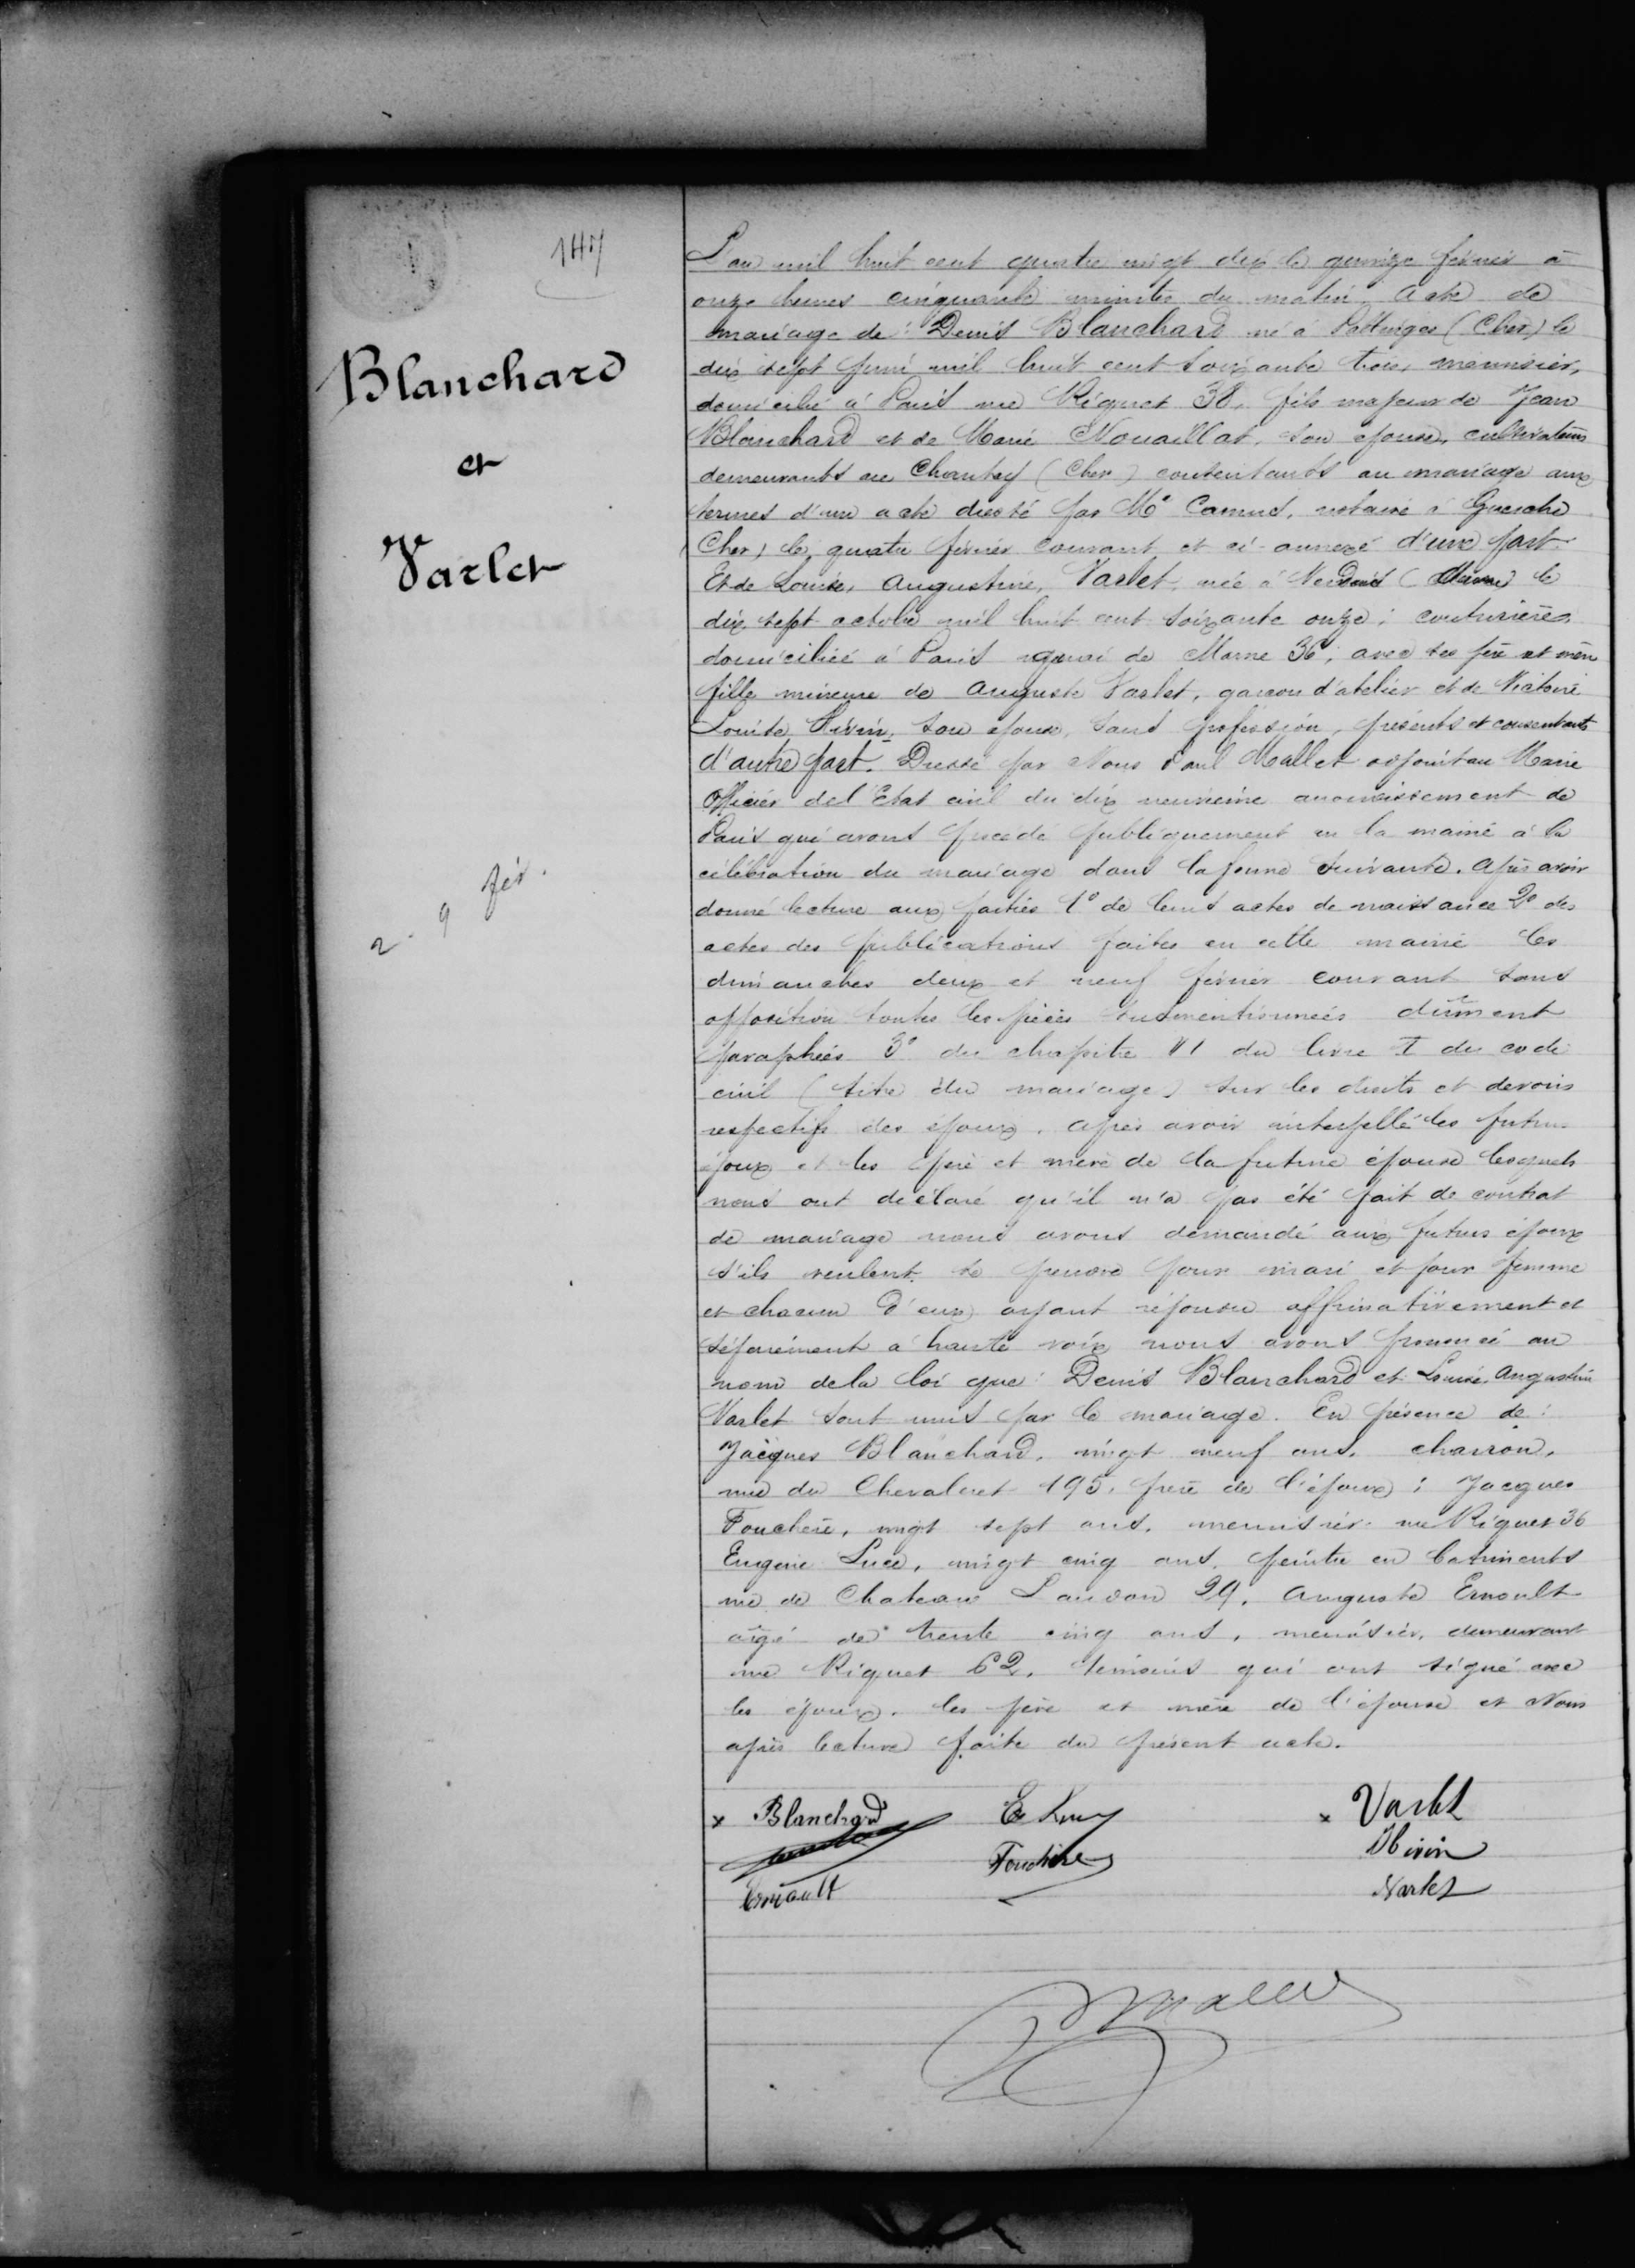147
Blanchard
et
Varlet
L'an mil huit cent quatre vingt dix le quinze février à
onze heures cinquante minutes du matin. Acte de
mariage de Denis Blanchard né à Pattunges (Cher) le
dix sept juin mil huit cent soixante trois, menuisier,
domicilié à Paris rue Réquet 38. fils majeur de Jean
Blanchard et de Marie Nouallat, son épouse, cultivateurs
demeurant au Chantey (Cher) consentants au mariage aux
termes d'un acte dressé par Me Camud, notaire à Guesche
(Cher) les quatre février courant et si-annexé d'une part.
Et de Louise, Augustine, Varlet, née à Nerdand (Marne) le
dix sept octobre mil huit cent soixante onze: couturière
domiciliée à Paris quai de Marne 36; avec ses père et mère
fille mineure de Auguste Varlet, garçon d'atelier et de Victoire
Louise Hivin, son époux, sans profession, présents et consentants
d'autre part. Dressé par Nous Paul Mallet adjoint au Maire
Officier de l'Etat civil du dix neuvième arrondissement de
Paris qui avons procédé publiquement en la mairie à la
célébration du mariage dans la forme suivante. après avoir
donné lecture aux parties 1° de leurs actes de naissance 2° des
actes de publications faites en cette maire les
dimanches deux et neuf février courant sans
opposition toutes les pièces susmentionées dûment
paraphées 3° du chapitre VI du livre 1 du code
civil (titre du mariage) sur les droits et devoirs
respectifs des époux. après avoir interpellé les futurs
poux et les père et mère de la future épouse lesquels
nous ont déclaré qu'il n'a pas été fait de contrat
de mariage nous avons demandé aux futurs époux
s'ils veulent se prendre pour mari et pour femme
et chacun d'eux ayant répondu affirmativement et
séparément à haute voix nous avons prononcé au
nom de la loi que: Denis Blanchard et Louise Augustine
Varlet sont unis par le mariage. En présence de:
Jacques Blanchard, vingt neuf ans, charron,
rue du Chevaleret 195,frère de l'épouse: Jacques
Fouchère, vingt sept ans, menuisier, rue Riquet 36
Eugénie Luce, vingt cinq ans, peintre en batiments
rue du Chateau Laudan 29, Auguste Ernoult
âgé de trente cinq ans, menuisier, demeurant
rue Riquet 62, témoins qui ont signé avec
les époux, les père et mère de l'épouse et Nous
après lecture faite du présente acte.
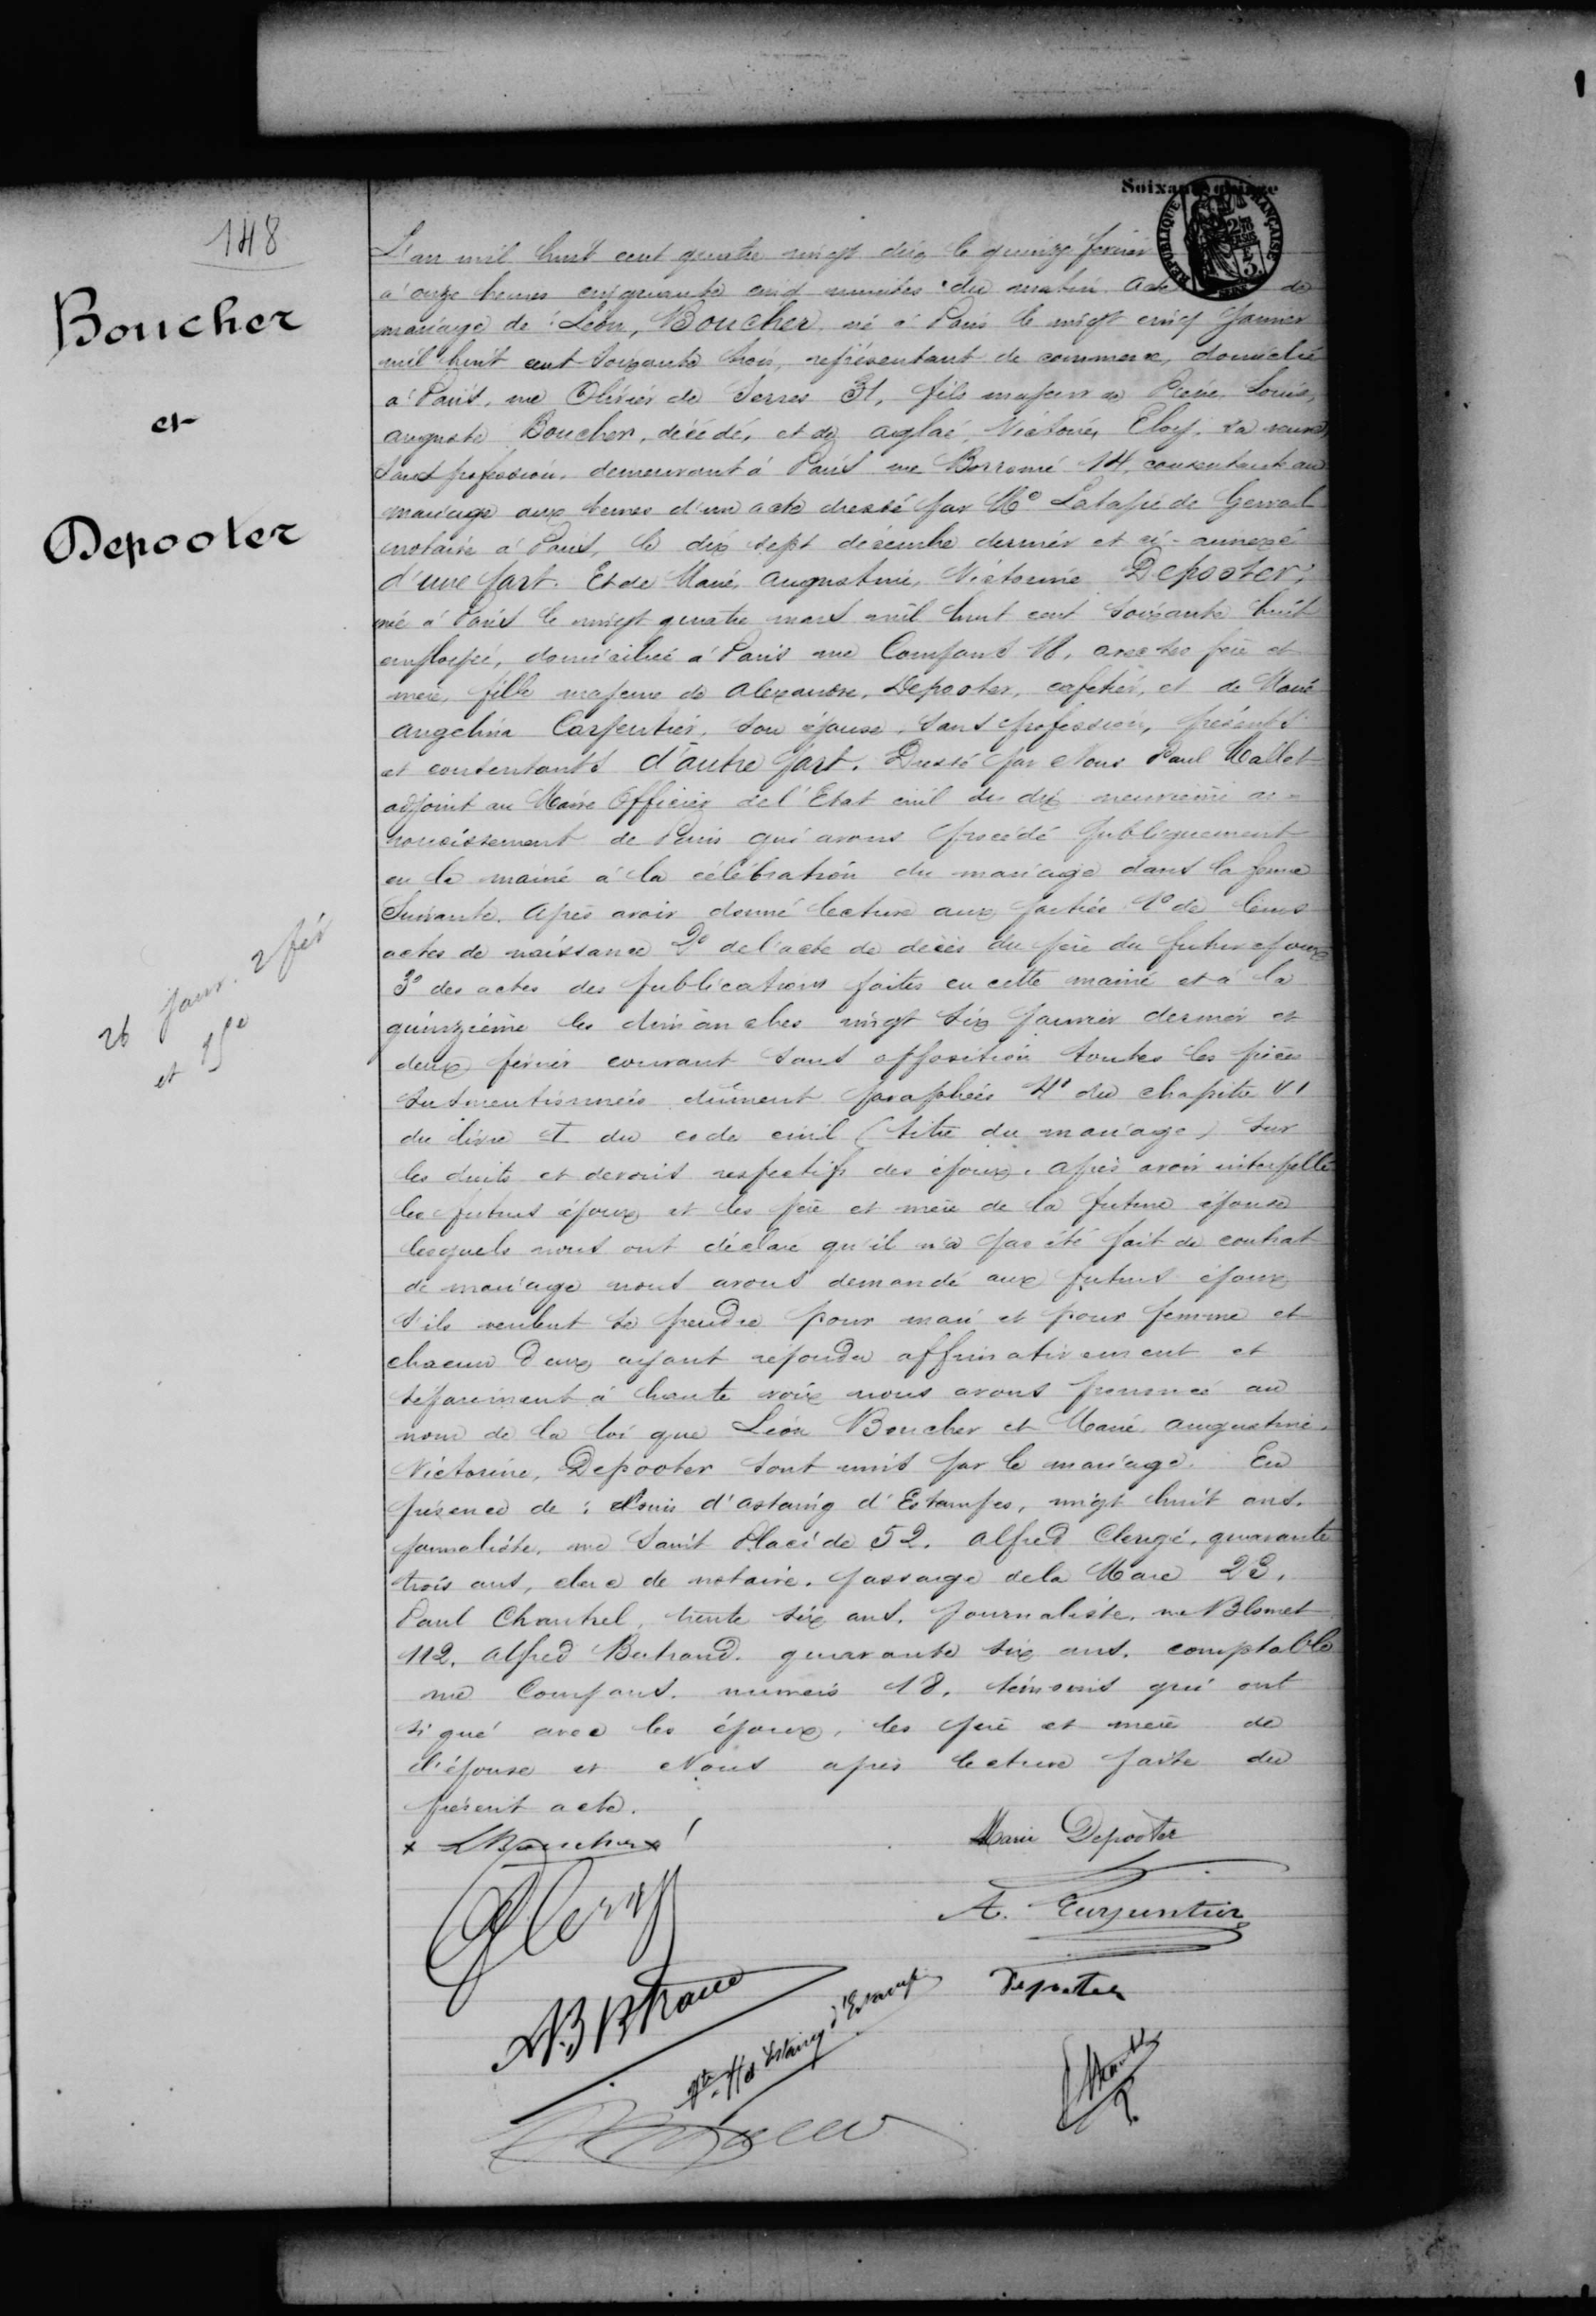148
Boucher
et
Depooler
L'an mil huit cent quatre vingt dix le quinze février
à onze heures cinquante cinq minutes du matin acte de
mariage de Léon, Boucher, né à Paris le vingt cinq Janvier
mil huit cent soixante trois, représentant de commerce, domicilié
à Paris, rue Olivier de Serres 31, fils majeur de René Louis,
auguste Boucher, décédé, et de aglaé, Victoire Eloy, sa veuve,
sans profession, demeurant à Paris rue Borroné 14, consentant au
mariage aux heures d'un acte dressé par M° Latapi de Gerval
notaire à Paris le dix sept décembre dernier et ci-annexé
d'une part. Et de Marie, Augustine, Victorine, Depooler,
né à Paris le vingt quatre mars mil huit cent soixante huit
employé, domiciliée à Paris rue Confand 18, avec ses père et
mère, fille majeure de Alexandre Depooler, cafetier, et de Nous
angelina Carpentier, son épouse, sans profession, présents
et consentants d'autre part. Dressé par Nous Paul Mallet
adjoint au Maire Officier de l'Etat civil du dix-neuvième ar-
rondissement de Paris qui avons procédé publiquement
en la mairie à la célébration du mariage dans la forme
suivante. après avoir donné lecture aux parties 1° de leurs
actes de naissance 2° de l'acte de décès du père du futur epoux
3° des actes des publications faites en cette mairie et à la
quinzième les dimanches vingt six janvier dernier et
deux février courant sans opposition toutes les pièces
susmentionnées dûment paraphées 4° du chapitre VI
du livre I du code civil (titre du mariage) sur
les droits et devoirs respectifs des époux. après avoir interpellé
les futurs époux et les père et mère de la future épouse
lesquels nous ont déclaré qu'il n'a pas été fait de contrat
de mariage nous avons demandé aux futurs époux
s'ils veulent se prendre pour mari et pour femme et
chacun d'eux ayant répondu affirmativement et
séparément à haute voix nous avons prononcé au
nom de la loi que Léon Boucher et Marie augustine
Victoire, Depooler sont unis par le mariage. En
présence de: Louis d'astaing d'Estampes vingt huit ans,
journalier, rue Saint Placide 52. Alfred Clengé, quarante
trois ans, clerc de notaire, passage de la Mare 23,
Paul Chantrel, trente six ans, journalier, rue Blomet
112, Alfred Bertrand quarante six ans, comptable
rue Compans, numéro 18, témoins qui ont
signé avec les époux, les père et mère de
l'épouse et Nous après lecture faite du
présent acte.